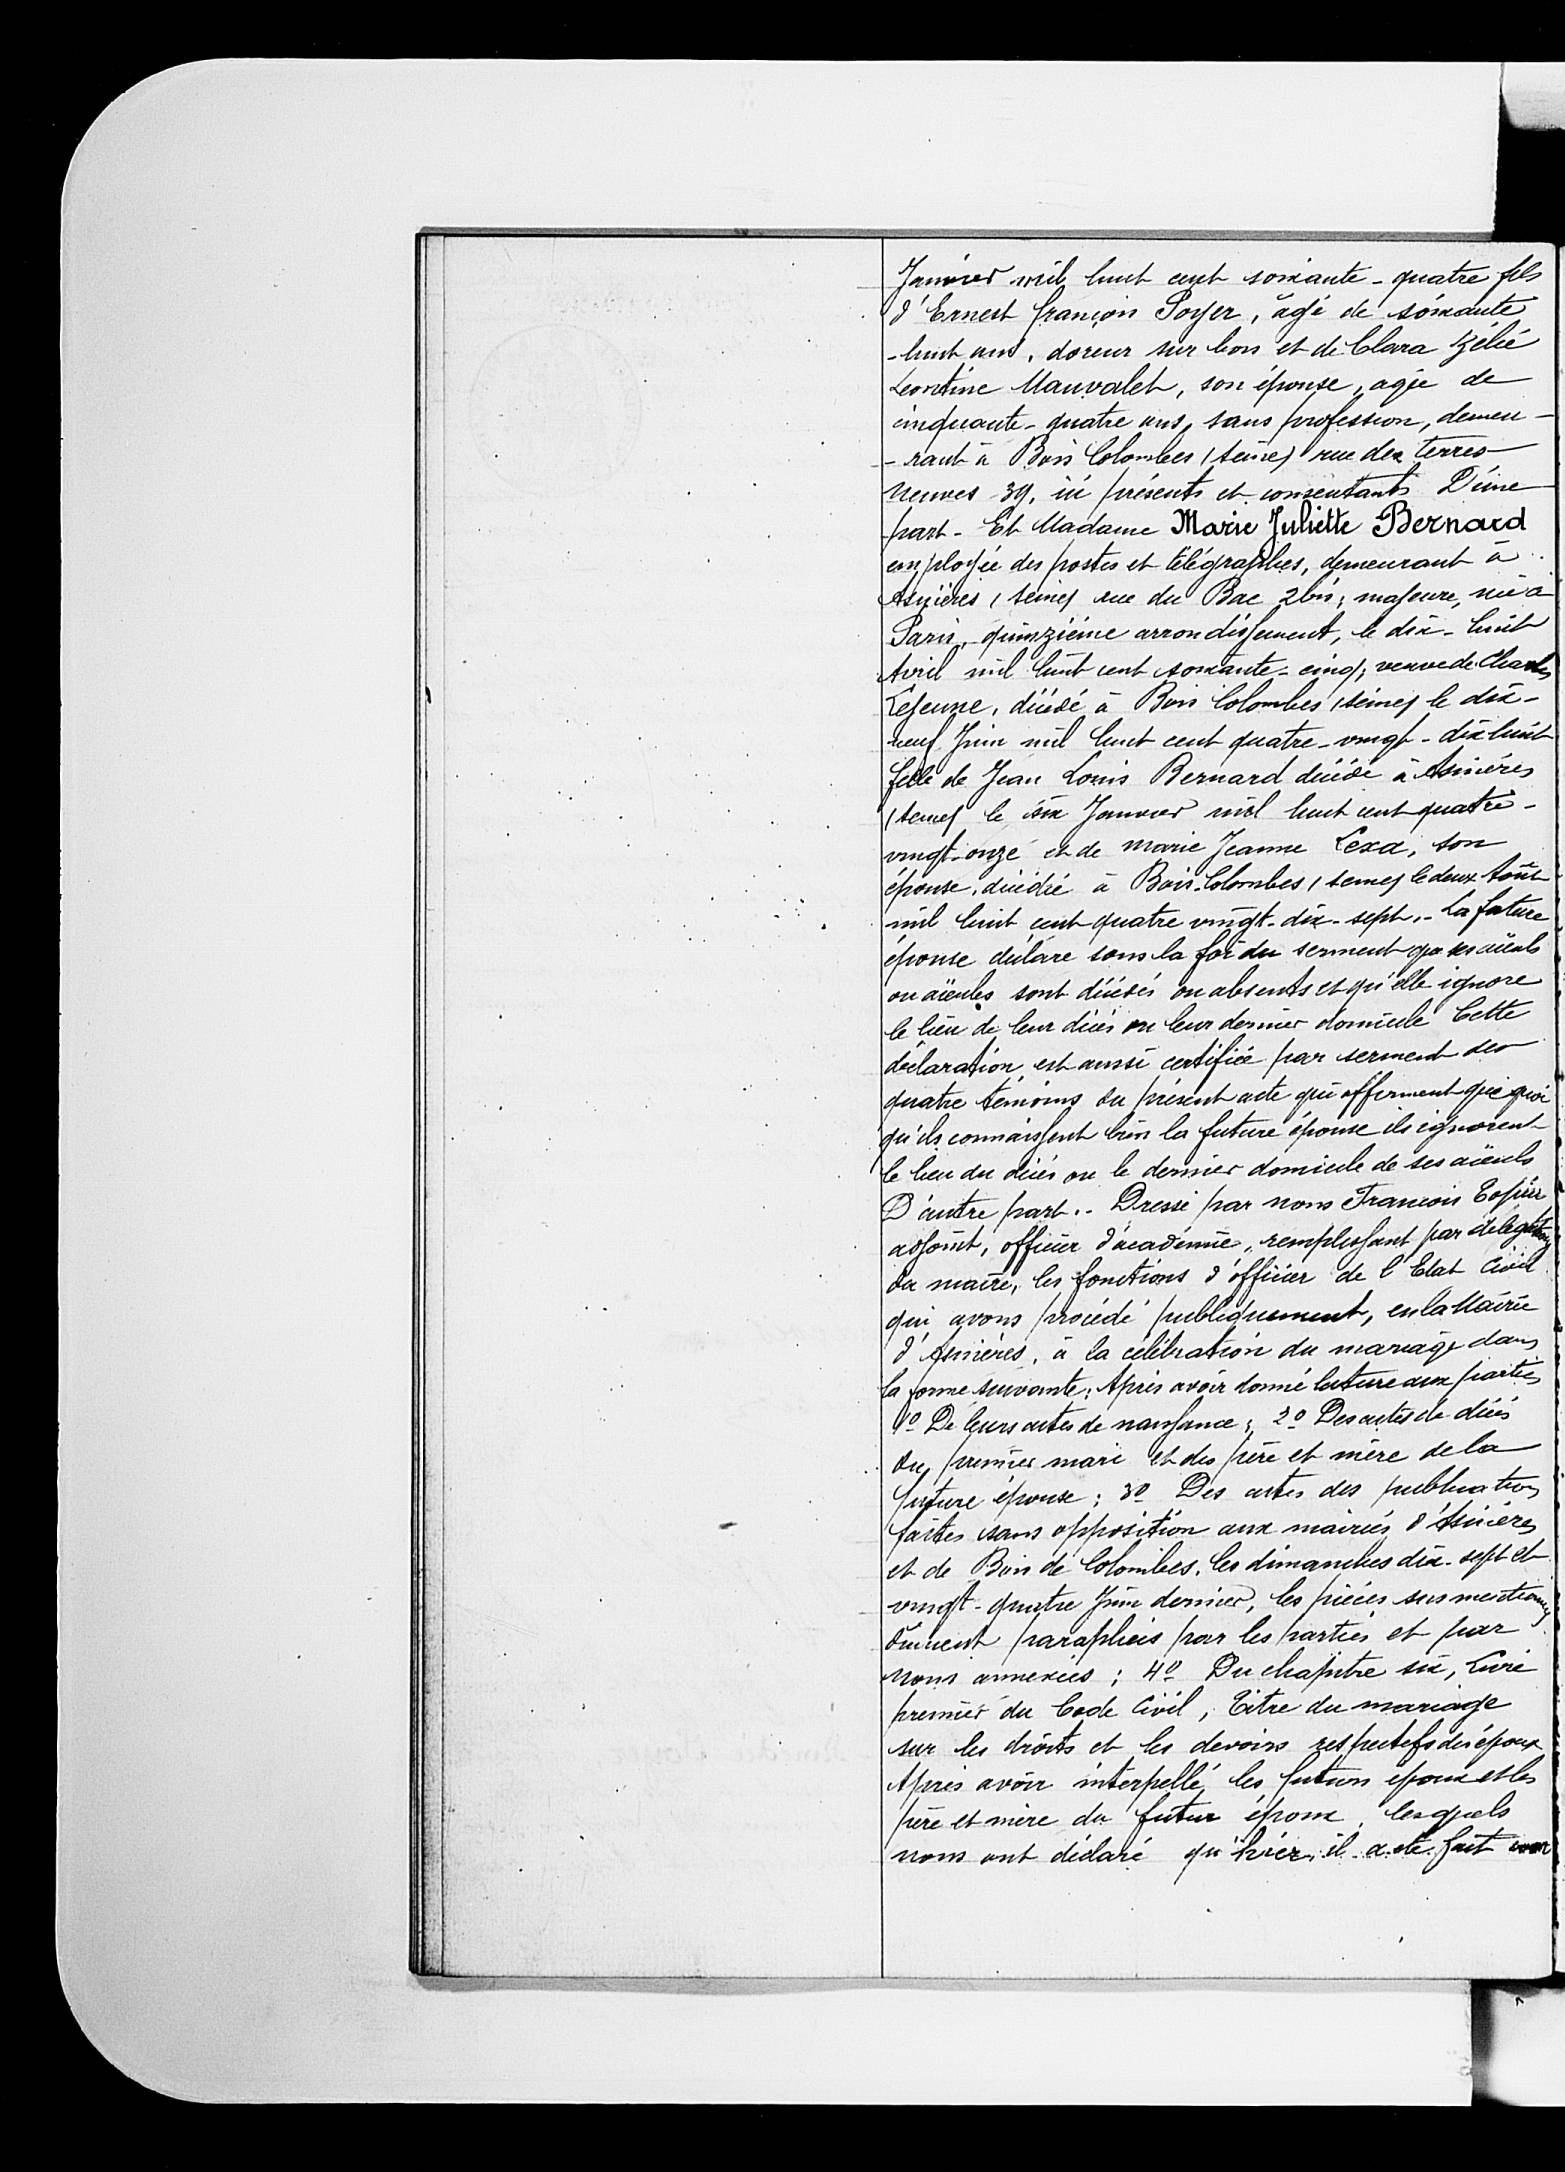Janvier mil huit cent soixante-quatre fils
d'Ernest françois Poyer âgé de soixante
-huit ans, doreur sur lons et de Elena Izélié
Léontine Mauvalet, son épouse, agée de
cinquante-quatre ans, sans profession, demeu-
-rant à Bois Colombes (Seine) rue des terres-
neuves 39, ici présents et consentants D'une
part. Et Madame Marie Juliette Bernard
employée des postes et télégraphes, demeurant à
Asnières (Seine) rue du Bac, 2 bis, majeure, née à
Paris, quinzième arrondissement, le dix-huit
Avril mil huit cent soixante-cinq, veuve de Charles
Lejeune, décédé à Bois Colombes (Seine) le dix-
neuf Juin mil huit cent quatre-vingt-dix huit
fille de Jean Louis Bernard décédé à Asnières
(Seine) le six Janvier mil huit cent quatre-
vingt-onze et de marie Jeanne Lexa, son
épouse, décédée à Bois-Colombes (seine) le deux Août
mil huit cent quatre vingt-dix-sept.-La future
épouse déclare dans la foi du serment que ses aïeuls
ou aïeules sont décédés ou absents et qu'elle ignore
le lieu de leur décès ou leur dernier domicile Cette
déclaration est aussi codifiée par serment des
quatres témoins du présent acte qui affirment que quoi
qu'ils connaissent bien la future épouse ils ignorent
le lieu du décés ou le dernier domicile de ses aïeules
D'autre part.-Dressé par nous Francoi Capuir
adjoint, officier d'académie, remplafant par délégat
du maire, les fonctions d'officier de l'Etat Civil
qui avons procédé publiquement, en la Mairie
d'Asnières, à la célébration du mariage dans
la forme suivant: Après avoir donné lecture aux parties
1° De leurs actes de naifance: 2° Des actes de décédés
du premier mari et de père et mère de la
future épouse: 3° Des actes des publication
faites sans opposition aux mairies d'Asnières
et de Bois de Colombes, les dimanches dix-sept et
vingt-quatre Juin dernier, les pièces susmention
dûment paraphées par les parties et par
nous annexées; 4° Du chapitre six, Livre
premier du Code Civil, Titre du mariage
sur les droits et les devoirs respectifs des époux
Après avoir interpellé les futurs époux et les
père et mère du futur époux, lesquels
nous ont déclaré qu'hier il acte faut
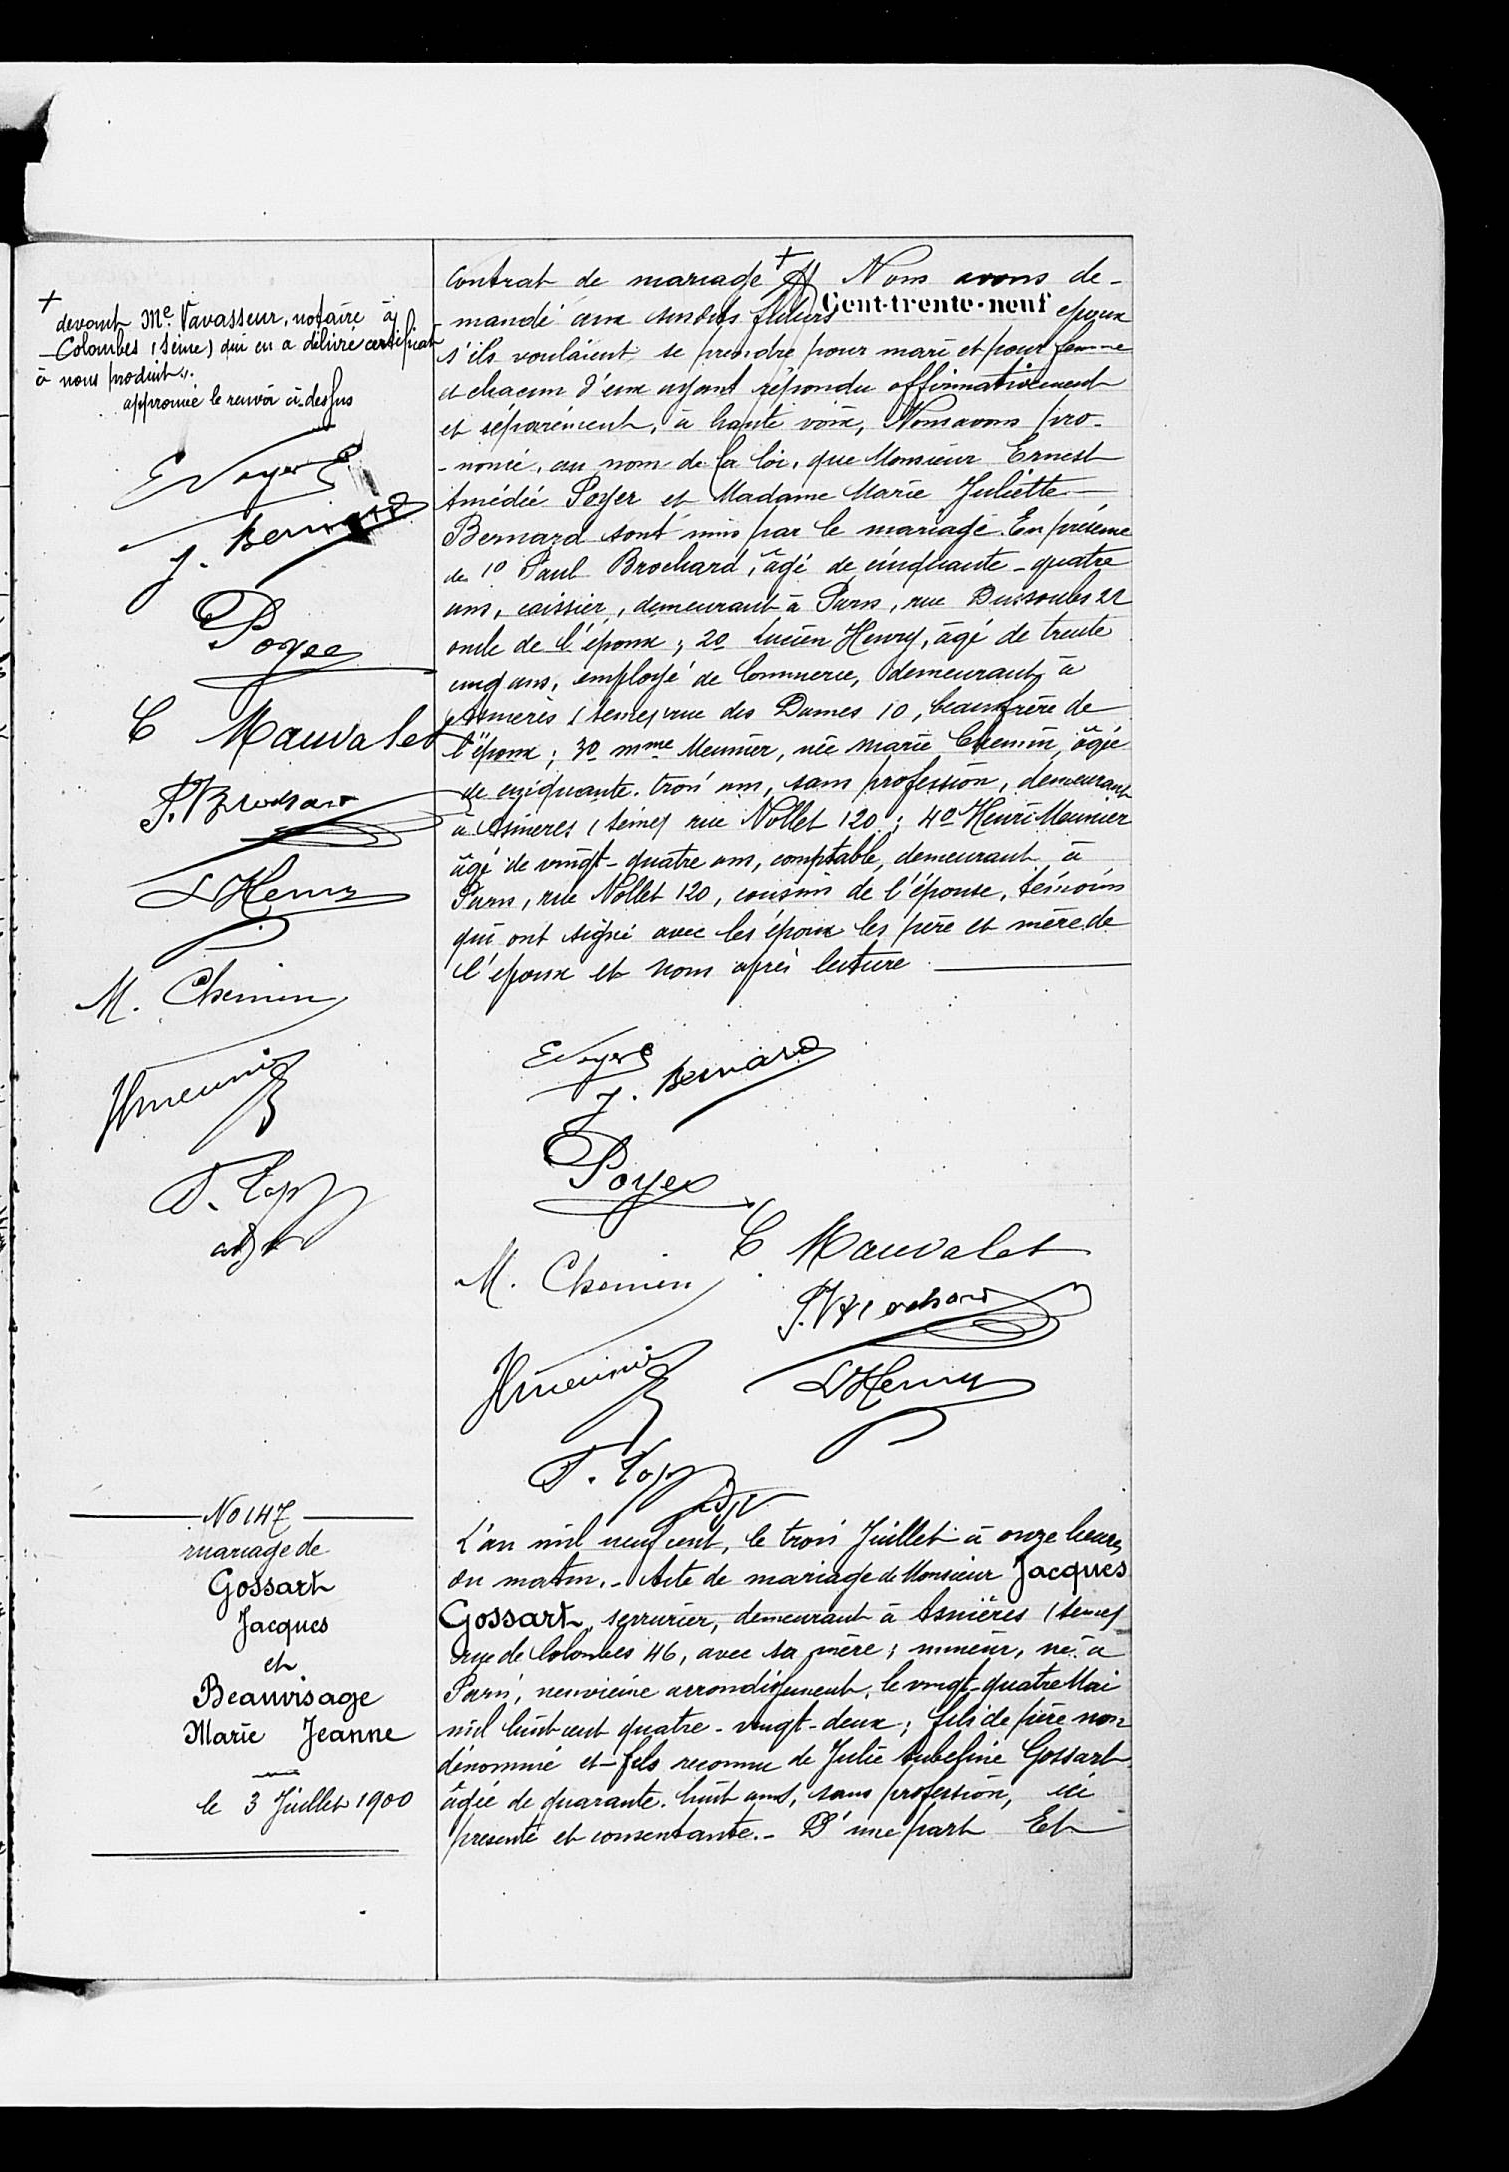contrat de mariage. Nous avons de-
mandé aux sondeds futurs époux
s'ils voulaient se prendre pour mari et pour femme
et chacun d'eux ayant répondu affirmativement
et séparément, à haute voix, Nous avons pro-
-noncé, au nom de la loi, que Monsieur Ernest
Amédée Poyer et Madame Marie Juliette
Bernard sont unis par le mariage En présence
de 1° Paul Brochard, âgé de cinquante quatre
ans, caissier, demeurant à Paris, rue Dussoubs 22
oncle de l'époux, 2° Lucien Henry, âgé de trente
cinq ans, employé de Commerce, demeurant à
Asnières (Seine) rue des Dunes 10, beaufrère de
l'époux; 3° mme Meunier, née Marie Crenin, âgée
de cinquante-trois ans, sans profession, demeurant
à Asnieres (Seine) rue Nollet 120; 4° Henri Meunier
âgé de vingt-quatre ans, comptable, demeurant à
Paris, rue Nollet 120, cousin de l'épouse, témoins
qui ont signé avec les époux les père et mère de
l'époux et nous après lecture -
No 147
mariage de
Gossart
Jacques
et
Beauvisage
Marie Jeanne
le 3 juillet 1900
L'an mil neuf cent, le trois Juillet à onze heures
du matin, Acte de mariage de Monsieur Jacques
Gossart, serrurier, demeurant à Asnières (Seine)
rue de Colombes 46, avec sa mère; mineur, né a
Paris, neuvième arrondissement, le vingt quatre Mai
mil huit cent quatre-vingt-deux; fils de père non
dénommé et fils reconnu de Julie Adeline Gossart
âgée de quarante huit ans, sans profession, ici
présenté et consentant. D'une part Et